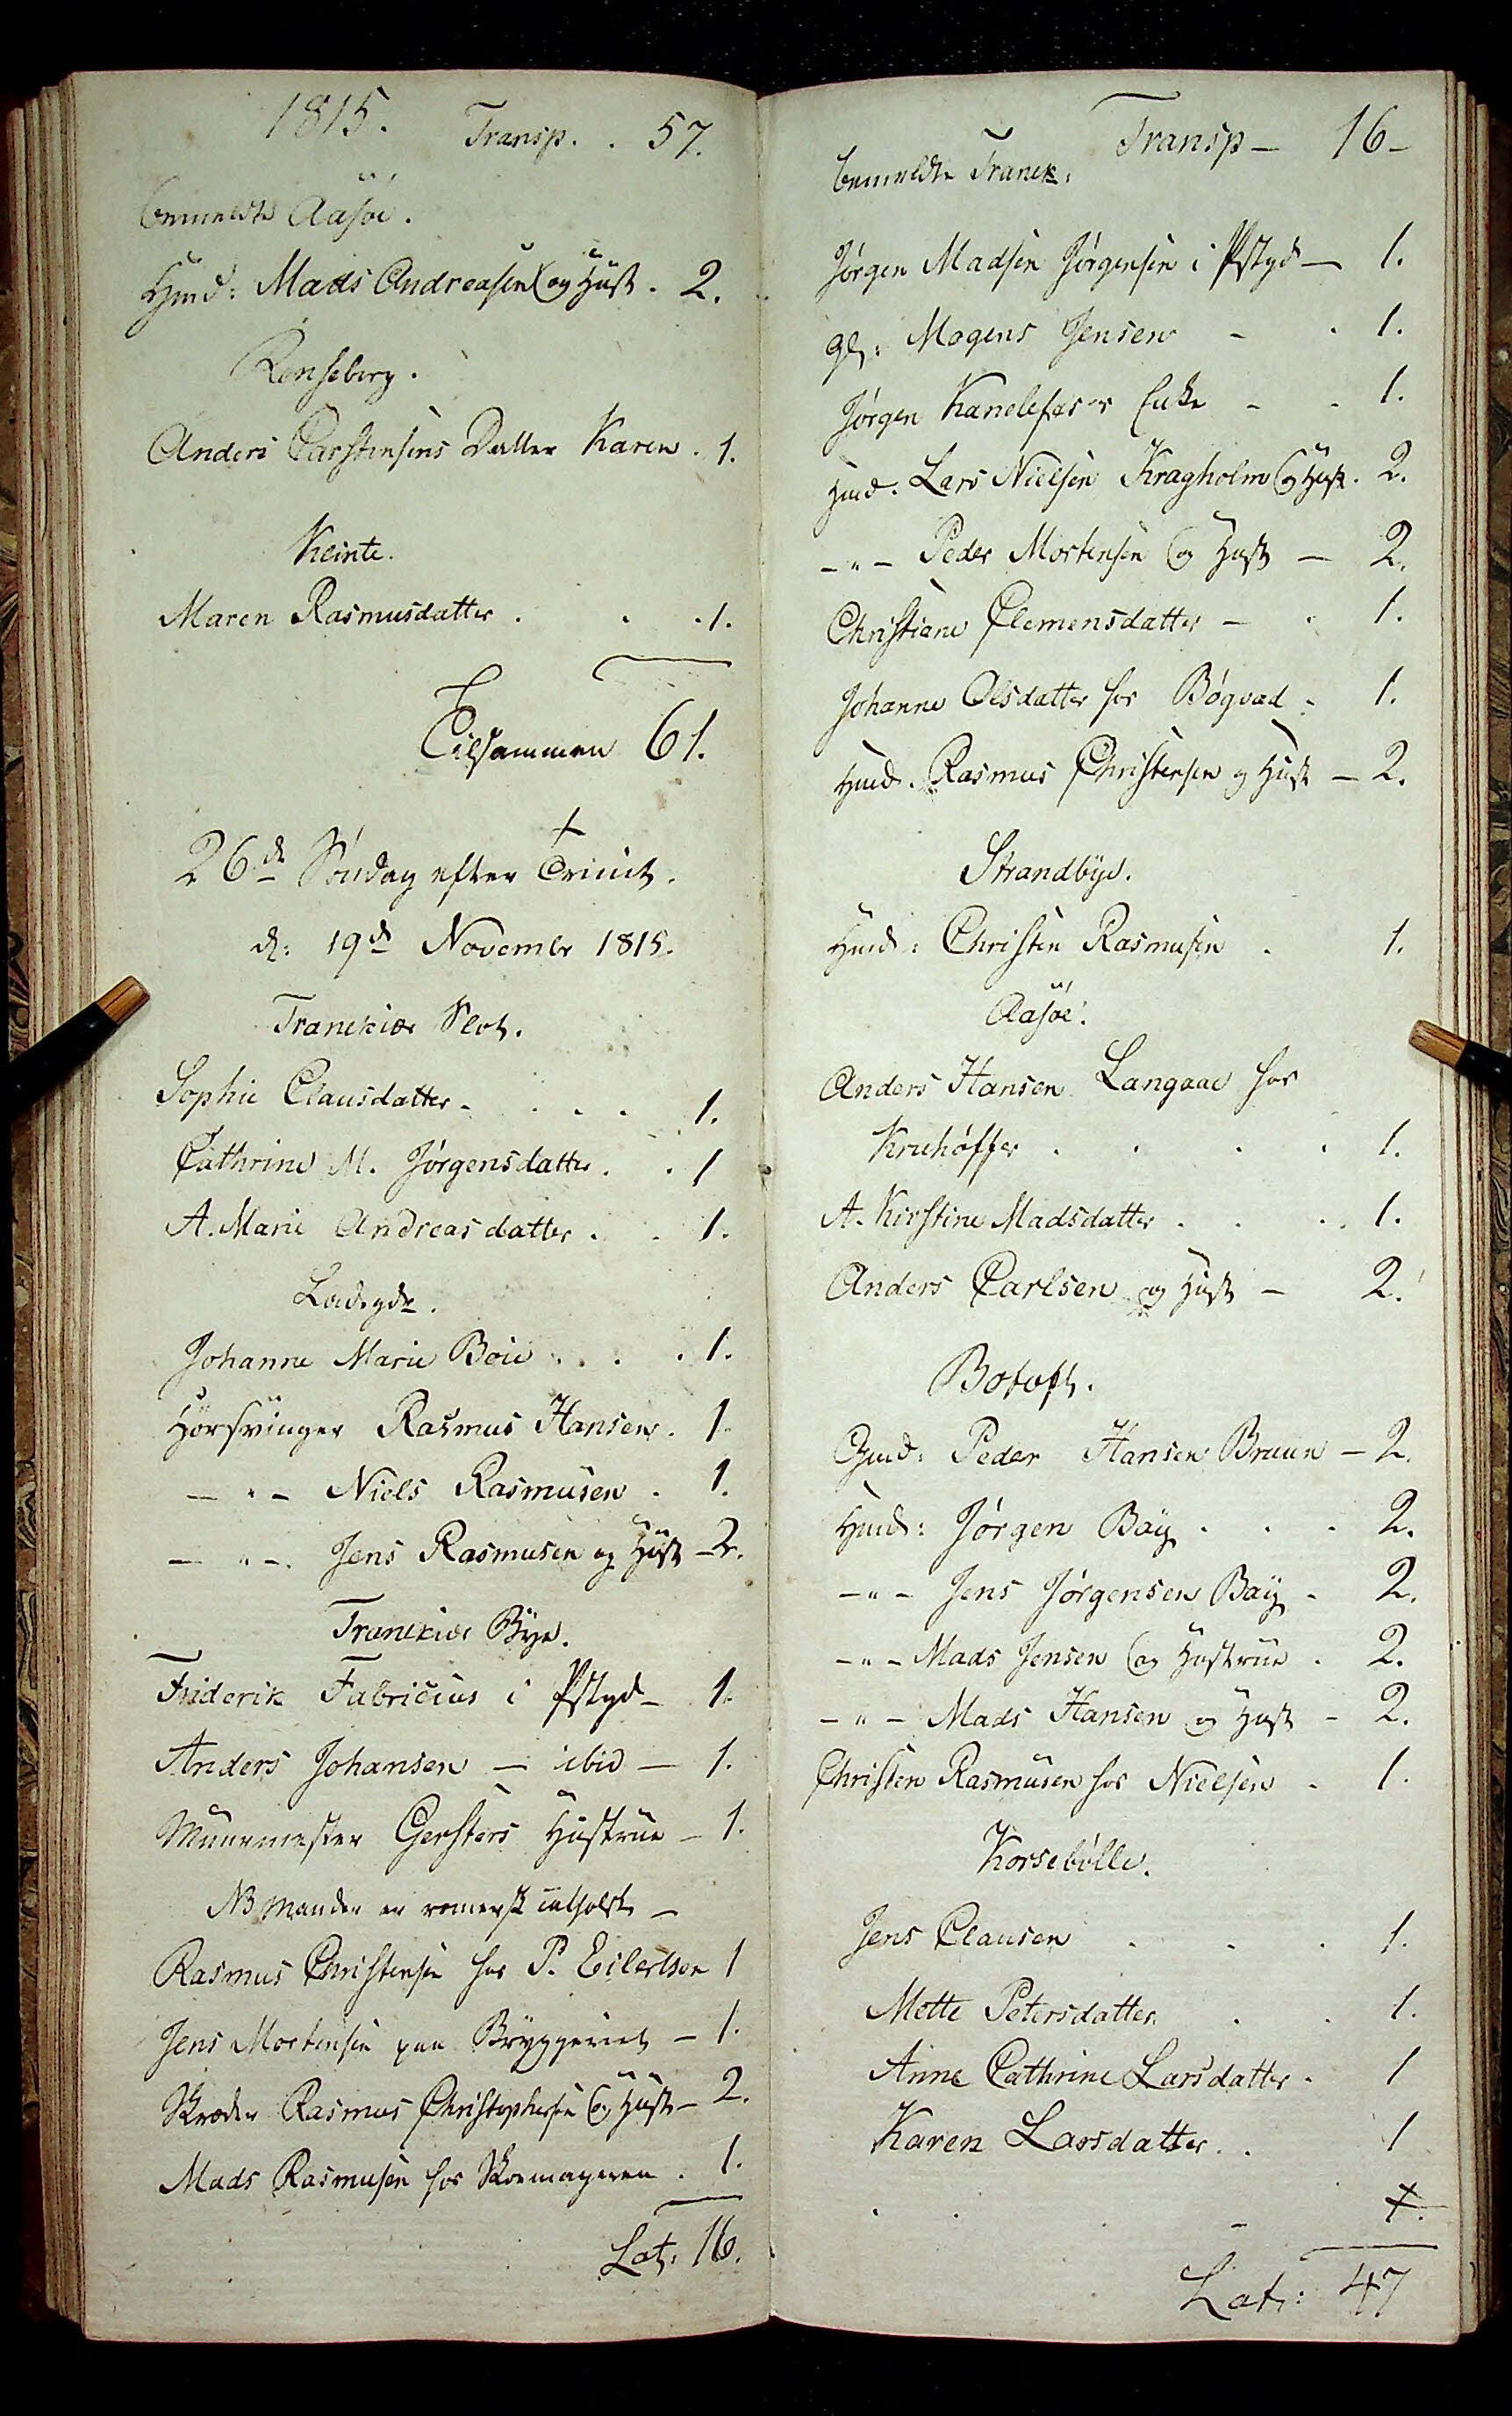1815.
Transp . . 57.
bemeldte Aasøe.
Hmd: Mads Andreasen og Hust . 2.
Renseberg.
Anders Carstensens Datter Karen . 1.
Klinte.
Maren Rasmusdatter . . . 1.
Tilsammen 61.
26de Søndag efter Trinit.
d: 19de Novembr 1815.
Tranekiær Slot.
Sophie Clausdatter . . . . 1.
Cathrine M. JørgensDatter . . 1.
A. Marie Andreasdatter . .1.
Ladegdr.
Johanne Marie Boie . . . . 1.
Hørsvinger Rasmus Hansen . 1.
-"- Niels Rasmusen . 1.
-"- Jens Rasmusen og Hustr - 2.
Tranekiær Bye.
Friderik Fabricius i Pstgdr 1.
Anders Johansen - ibid - 1.
Muurmester Gersters Hustrue - 1.
NB Manden er remerst## culholdt## -
Rasmus Christensen hos P. Eilertsen 1
Jens Mortensen paa Bryggeriet - 1.
Skræder Rasmus Christophersen og Hust - 2.
Mads Rasmusen hos Skoemager . 1.
Lat: 16.
Transp - 16-
bemmeldte Tranek:
Jørgen Madsen Jørgensen i Pstgd - 1.
gl: Mogens Jensen - . 1.
Jørgen Kanelefas's i Enke - . 1.
Hmd. Lars Nielsen Kragholm og Hust . 2.
-"- Peder Mortensen og Hust - 2.
Christiane Clemensdatter - . 1.
Johanne Olsdatter fos Bøgvad . 1.
Hmd. Rasmus Christensen og Hust - 2.
Strandbye.
Hmd: Christen Rasmusen . 1.
Aasøe:
Anders Hansen Langaae hos
Kruhøffer . . . . 1.
A. Kirstine Madsdatter . . . . 1.
Anders Carlsen og Hust - 2.
Botoft.
Gmd: Peder Hansen Bruun - 2.
Hmd: Jørgen Bay . . . 2.
-"- Jens Jørgensen Bay . 2.
-"- Mads Jensen og Hustrue . 2.
-"- Mads Hansen og Hust - 2.
Christen Rasmusen hos Nielsen . 1.
Korsebølle.
Jens Clausen . . . 1.
Mette Petersdatter . . 1.
Anne Cathrine Larsdatter . 1 
Karen Larsdatter . . 1
Lat: 47
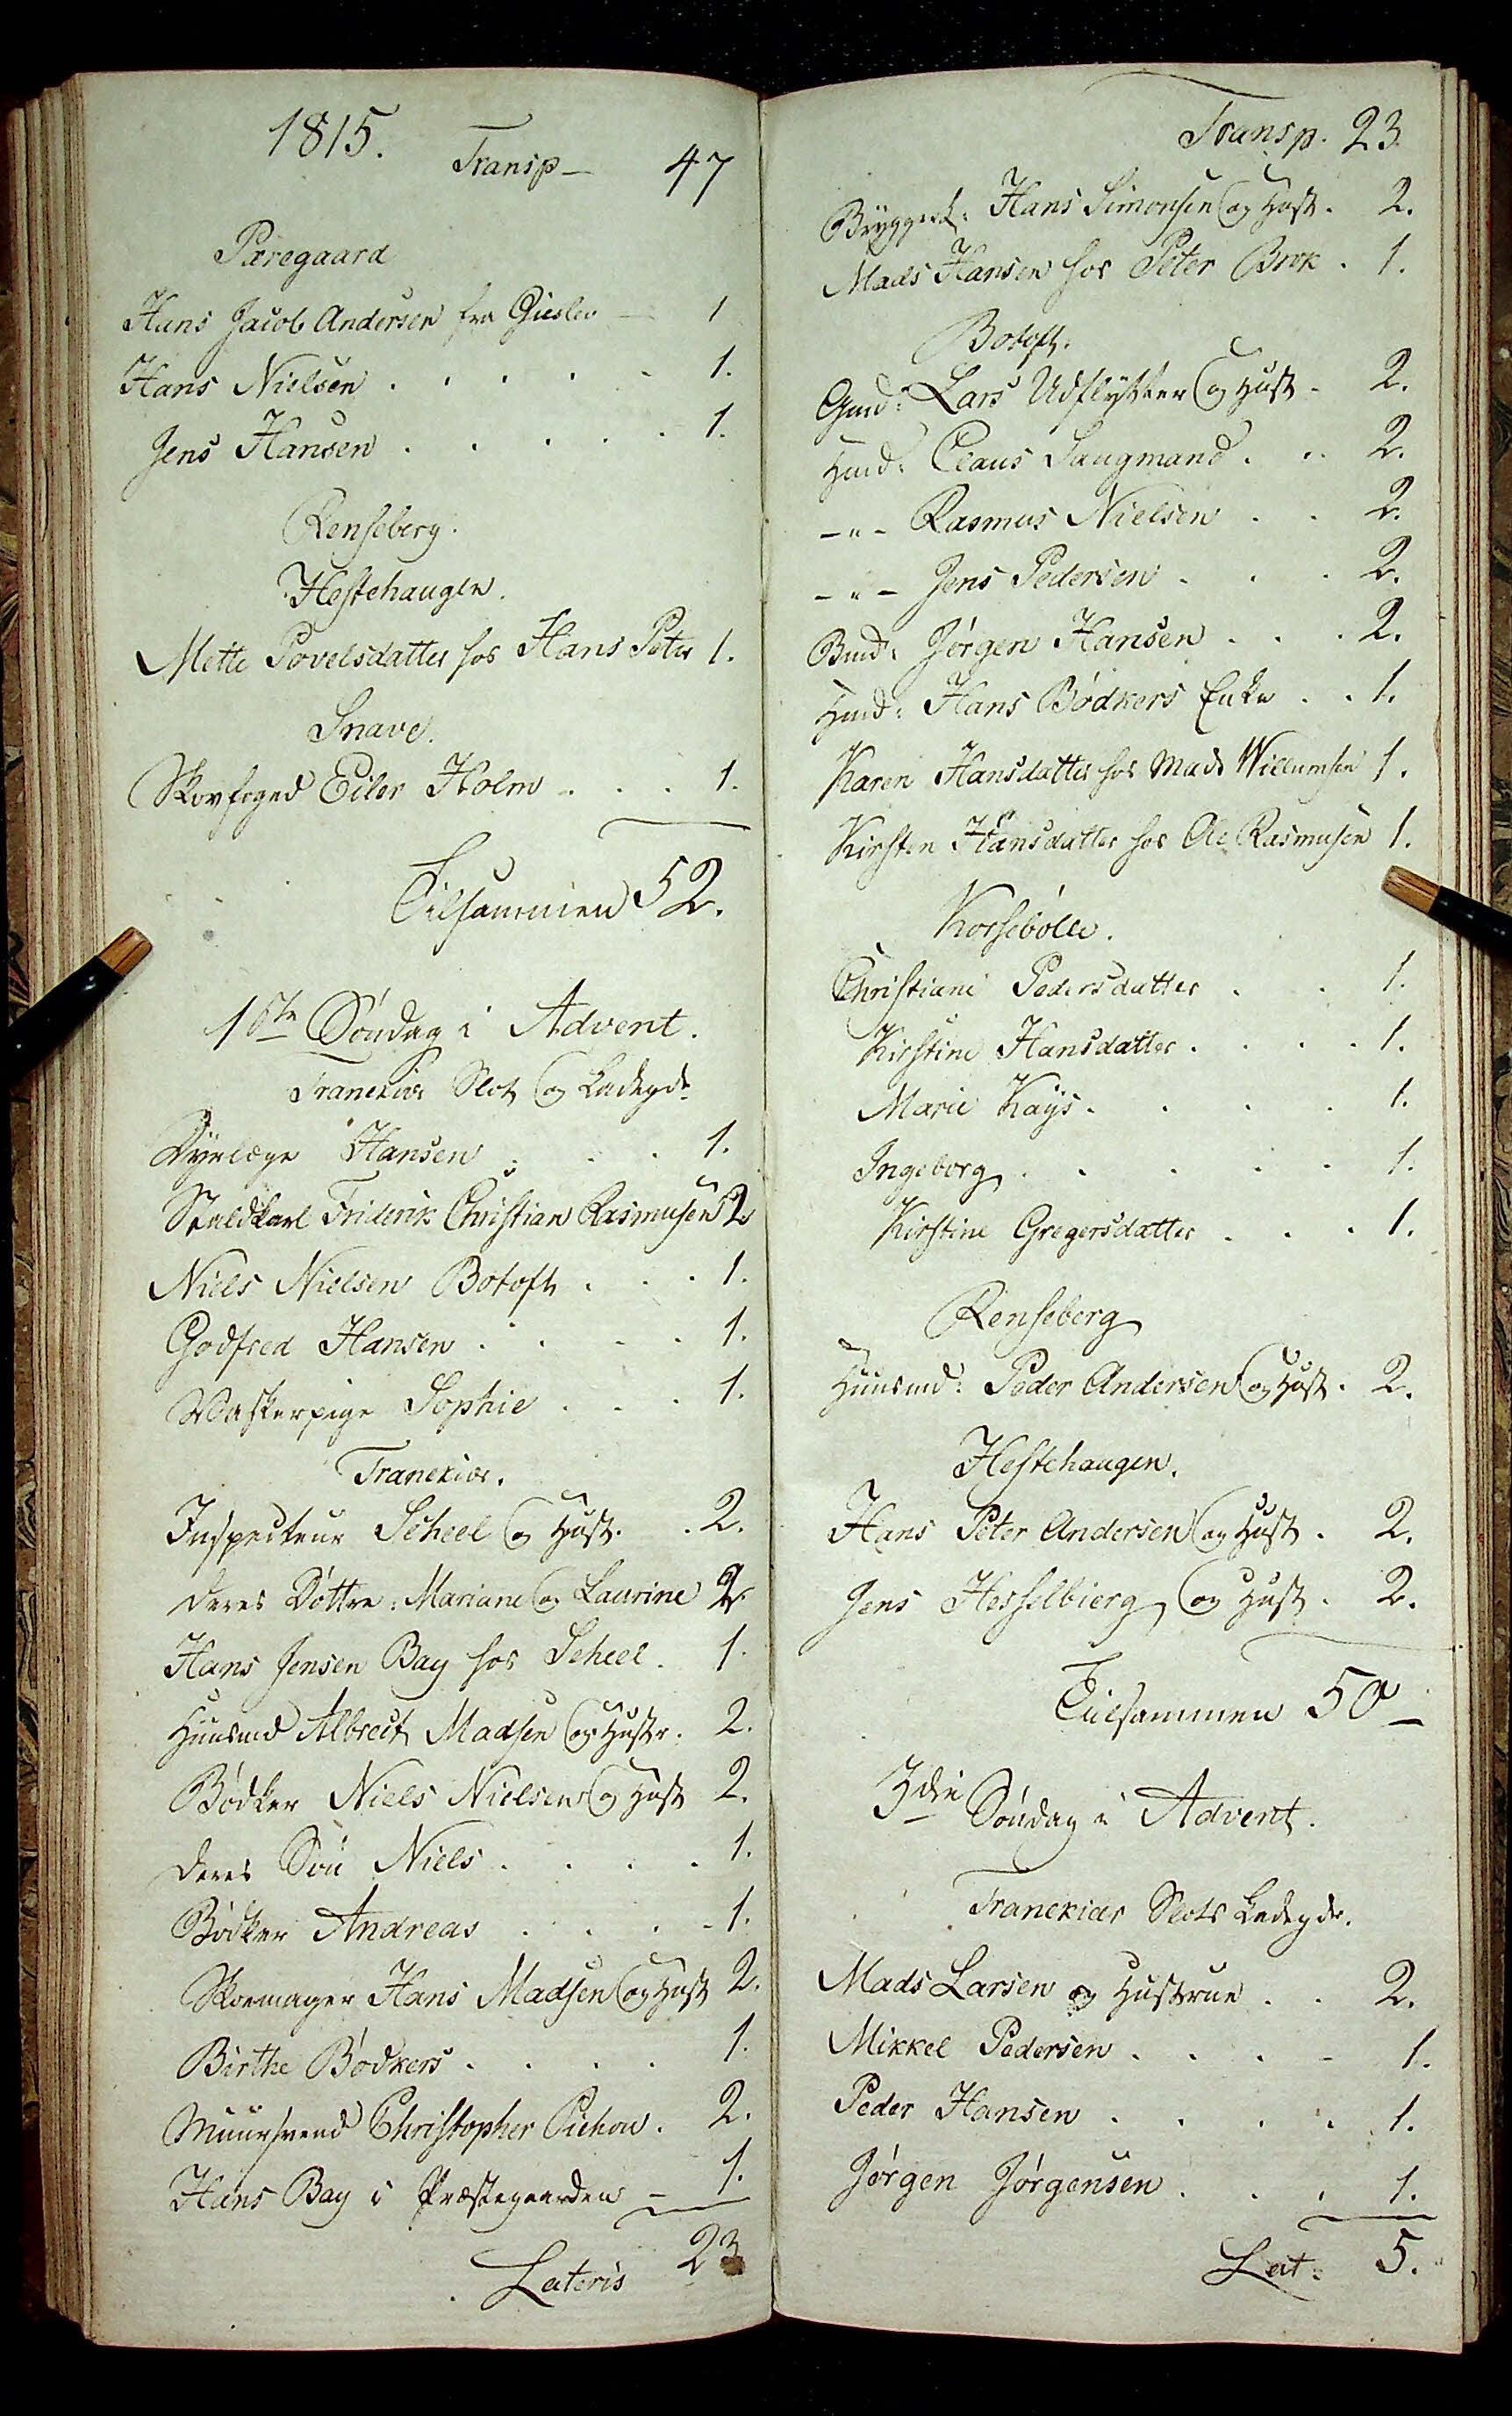1815.
Transp - 47
Pæregaard
Hans Jacob Andersen fra Gieslev - 1
Hans Nielsen  . . . . . 1.
Jens Hansen . . . . . 1.
Renseberg.
Hestehaugen
Mette Povelsdatter hos Hans Peter 1.
Snave.
Skofoged Eiler Holm . . . 1.
Tilsammen 52.
1ste Søndag i Advent.
Tranekiær Slot og Ladegdr
Dyrlæge Hansen . . . 1.
Staldkarl Friderik Christian Rasmusen 2.
Niels Nielsens Botoft . . . 1.
Godfred Hansen . . . . 1.
Vaskerpige Sophie . . . 1.
Tranekiær.
Inspecteur Scheel og Hust . . 2.
deres Døttre: Mariare og Laurine 2.
Hans Jensen Bay hos Scheel . 1
Huusmd Albrect Madsen og Hustr . 2.
Bødker Niels Nielsen og Hust 2.
deres Søn Niels . . . . 1.
Biødker Andreas . . . . 1.
Skoemager Hans Madsen og Hust 2.
Birthe Bødkers . . . . 1.
Muursvend Christopher Pichou . 2.
Hans Bay i Præstegaarden - 1.
Lateris 23
Transp. 23.
Bryggerk: Hans Simonsen og Hust . 2.
Mads Hansen hos Peter Brok . 1.
Botoft.
Gmd: Lars Udflytter og Hust . 2.
Hmd: Claus Saugmand . . 2.
-"- Rasmus Nielsen . . 2.
-"- Jens Pedersen . . . 2.
Bmd: Jørgen Hansen . . . 2.
Hmd: Hans Bødkers Enke . . 1.
Karen Hansdatter hos Mads Willumsen 1.
Kirsten Hansdatter hos Ole Rasmusen 1.
Korsebølle.
Christiane Pedersdatter . . 1.
Kirstine Hansdatter . . . . 1.
Marie Kays . . . . 1.
Ingeborg . . . . . 1.
Kirstine Gregersdatter . . . 1.
Renseberg
Huusmd: Peder Andersen og Hust . 2.
Hestehaugen.
Hans Peter Andersens og Hust . 2.
Jens Hesselbierg og Hust . 2.
Tilsammen 50-
3die Søndag i Advent.
Tranekiær Slots Ladegdr.
Mads Larsen og Hustrue . . 2.
Mikkel Pedersen . . . . 1.
Peder Hansen . . . . 1.
Jørgen Jørgensen . . . 1.
Lat: 5.